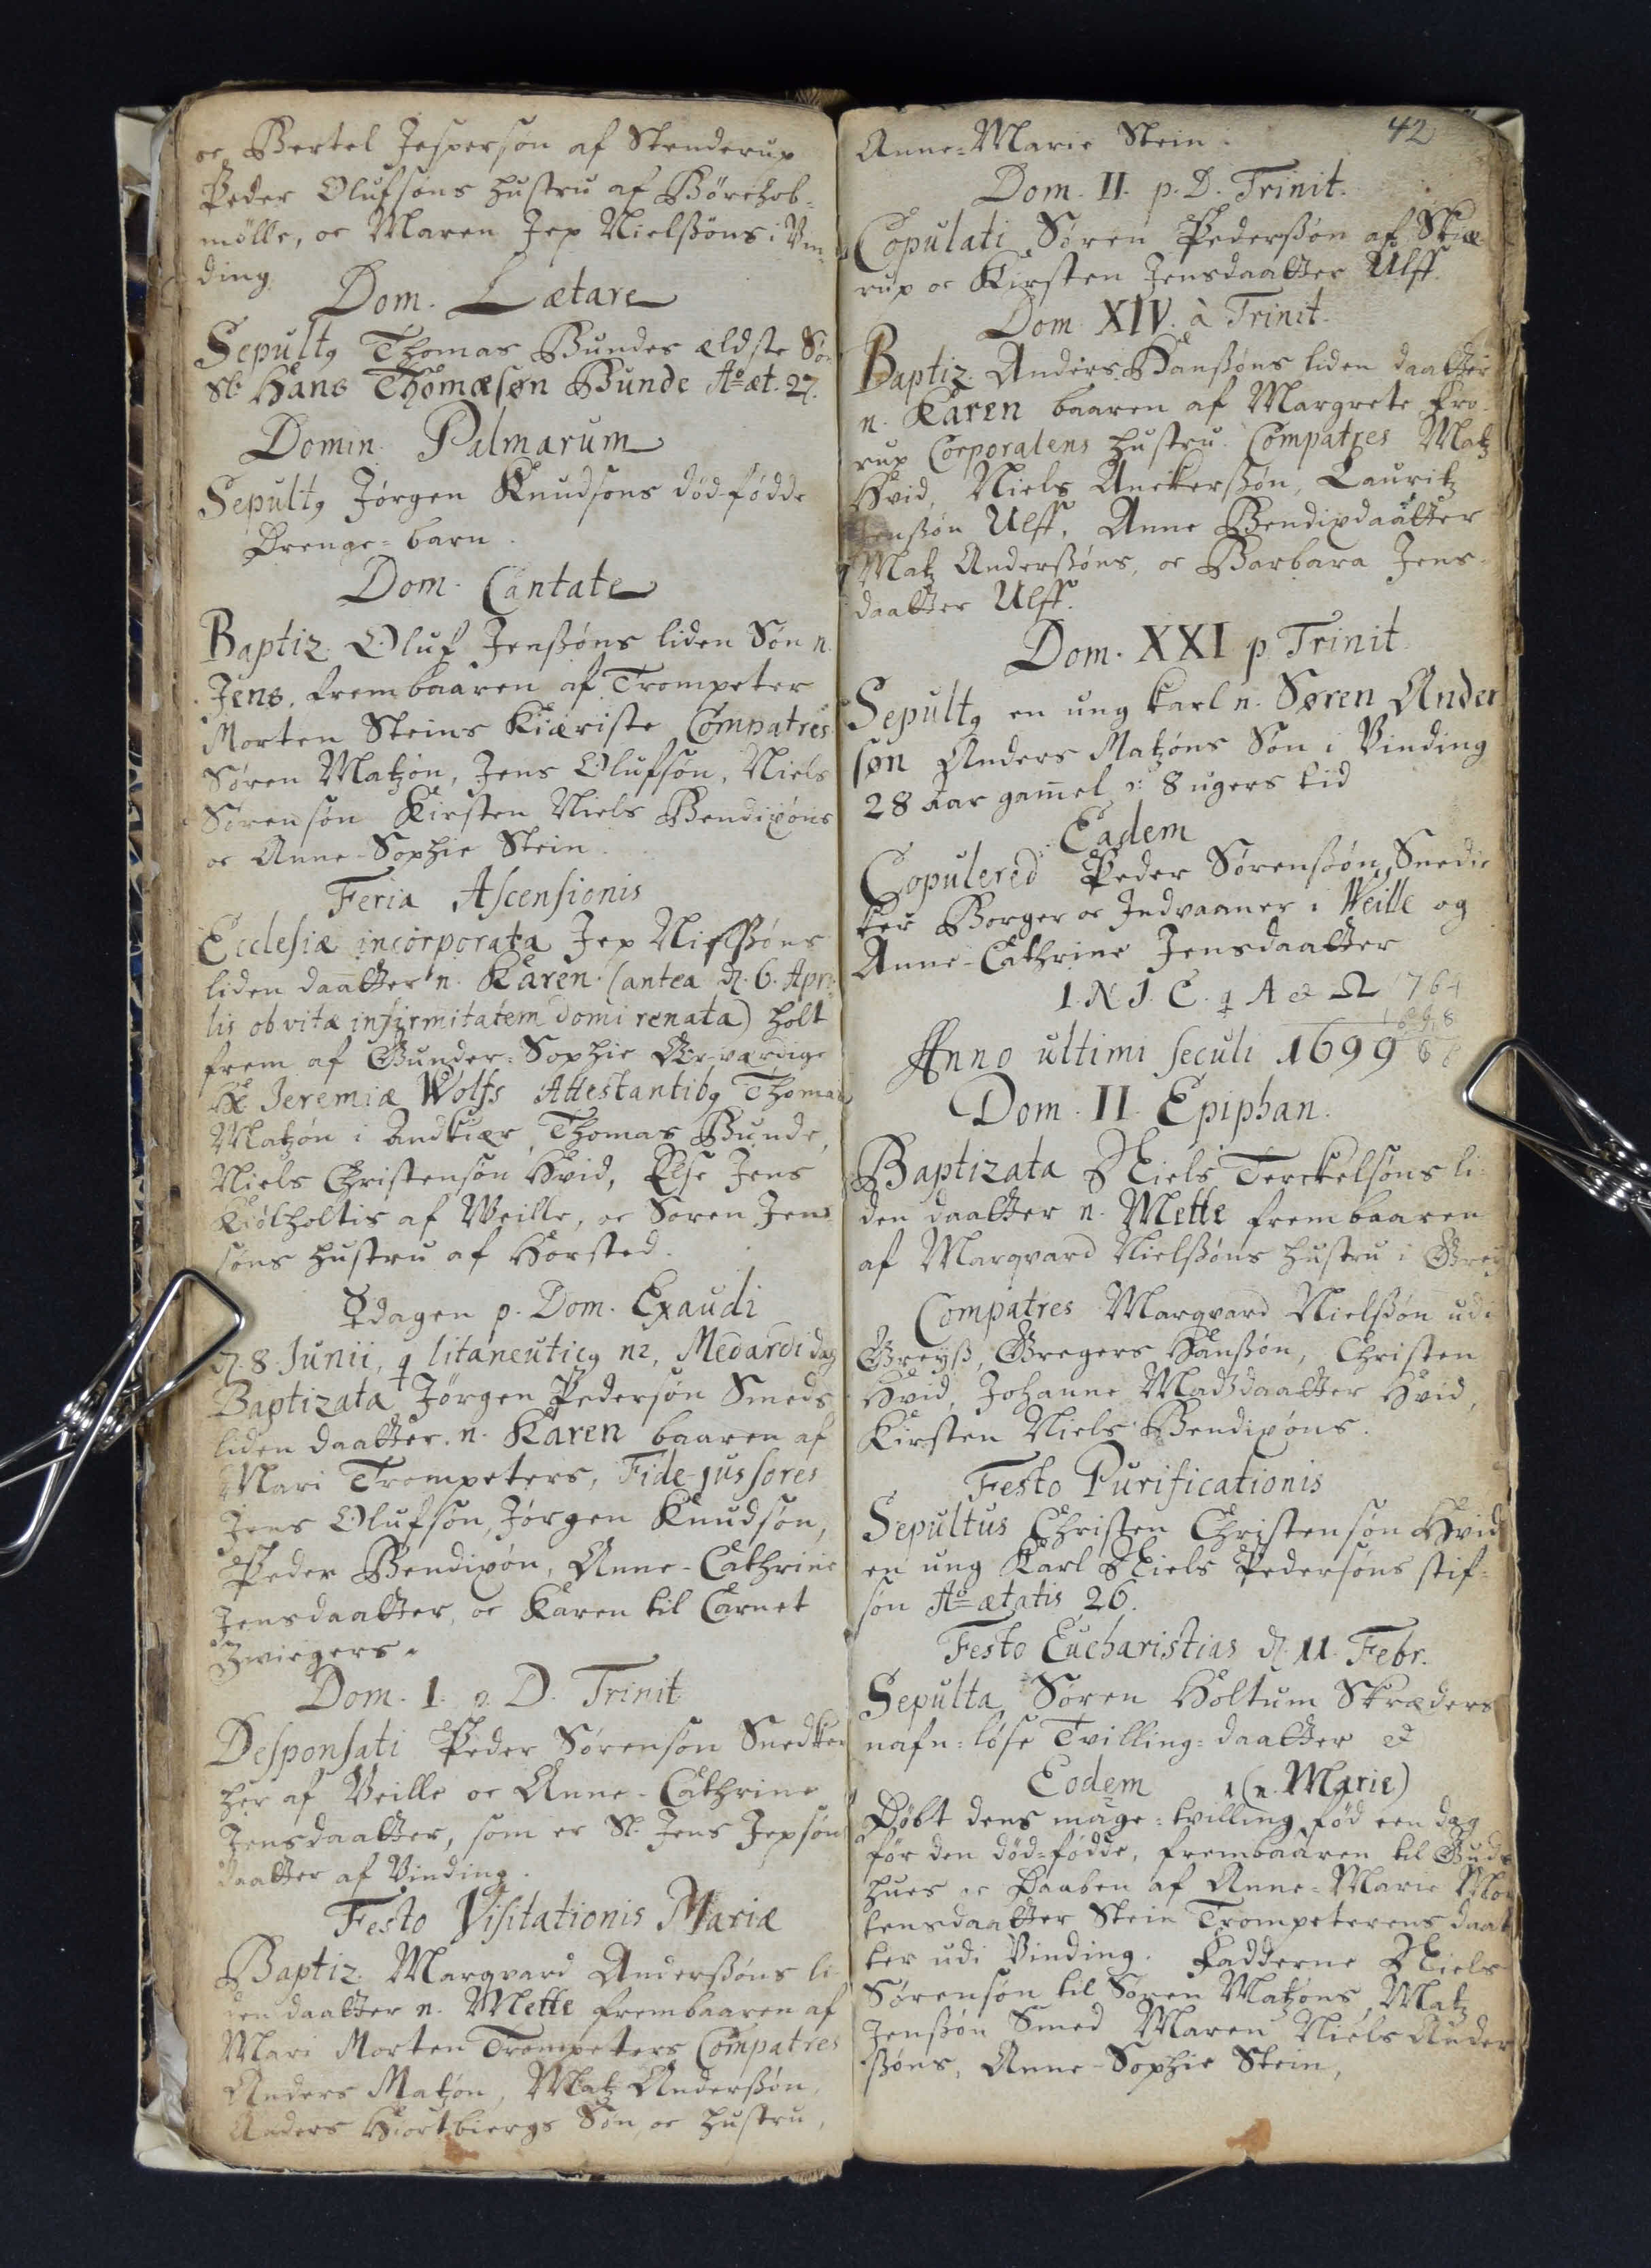oc Bertel Jespersøn af Stenderup
Peder Olufsøns hustru af Børchop¬
mølle, oc Maren Jep Nielssøns i Vin¬
ding
Dom. Læatre
Sepultq Thomas Bundes ældste Søn
Sl. Hans Thomasøn Bunde Ao æt. 27.
Domin Palmarum
Sepultq Jørgen Knudsøns død-fødde
Drenge=barn
Dom. Cantate
Baptiz. Oluf Jenssøns liden Søn n.
Jens, frembaaren af Trompeter
Morten Steins Kiæriste Compatres
Søren Matzøn, Jens Olufsøn, Niels
Sørensøn Kirsten Niels Bendixøns
oc Anne Sophie Stein
Feria Ascensionis
Ecclesiæ incorporata Jep Nissøns
liden daatter n. Karen. (antea d. 6 Apri¬
lis ob vitæ infirmitatem domi renata) holt
frem af Gunder=Sophie Ærværdige
Hl. Jeremiæ Wolfs Attestantibq Thoma 
Matzøn i Andkiær, Thomas Bunde
Niels Christensøn Hvid, Else Jens
Kiølholtis af Weille, oc Søren Jens
søns hustru af Horsted
☿ dagen p. Dom. Exaudi
d. 8 Junii. q litaneuticq nz, Medardi dag
Baptizata Jørgen Pedersøn Smeds 
liden daatter n. Karen, baaren af
Mari Trompeters, Fide- jussores
Jens Olufsøn, Jørgen Knudsøn
Peder Bendixøn, Anne- Cathrine
Jensdaatter, oc Karen til Cornet
Zvirgers.
Dom. I. p. D. Trinit
Desponsati Peder Sørensøn Snedker 
her af Veille oc Anne Cathrine 
Jensdaatter, som er Sl. Jens Jepsøns 
daatter af Vinding
Festo Visitationis Mariæ
Baptiz Marqvard Anderssøns li
den daatter n. Mette, frembaaren af
Mari Morten Trompeters Compatres
Anders Matzøn. Matz Anderssøn.
Anders Hiortbiergs Søn oc hustru
42
Anne=Marie Stein
Dom. II. p. D. Trinit.
Copulati Søren Pederssøn af Skiæ¬
rup oc Kirsten Jensdaatter Ulff.
Dom. XIV. a Trinit.
Baptiz Anders Hanssøns liden daatter
n. Karen, baaren af Margrete Fro¬
rup Corporalens hustru. Compatres Matz
Hvid, Niels Anckerssøn, Lauritz
Ulff, Anne Bendixdaatter
Matz Anderssøns, oc Barbara Jens
daatter Ulff
Dom. XXI p. Trinit
Sepultq en ung karl n. Søren Ander
søn, Anders Matzøns Søn i Vinding
28 aar gamel ↄ: 8 ugers tid
Eadem
Copulered Peder Sørenssøn Snedic
ker Borger oc Indvaaner i Weille og
Anne-Cathrine Jensdaatter
I. N. J. C. q A et Ω
Anno ultimi seculi 1699
Dom. II. Epiphan.
Baptizata Niels Terckelsøns li¬
den daatter n. Mette frembaaren
af Marqvard Nielssøns hustru i Grey 
Compatres Marqvard Nielssøn udi
Greyss, Gregers Hanssøn, Christen
Hvid, Johanne Madtzdaatter Hvid
Kirsten Niels Bendixøns
Festo Purificationis
Sepultus Christen Christensøn Hvid
en ung Karl Niels Pedersøns stif¬
søn Ao ætatis 26.
Festo Eucharistias d. 11. Febr.
Sepulta Søren Holtum Skræders
nafn=løse Tvilling=daatter et
Eodem. ## (n. Marie)
Døbt dens mage=tvilling, fød een dag
før den død=fødde, frembaaren
hues oc Daaben af Anne Marie
tensdaatter Stein Trompeterens daat
ter udi Vinding. Fadderne. Niels
Sørensøn til Søren Matzøns, Matz
Jenssøn Smed Maren Niels Ander
ssøns, Anne-Sophie Stein